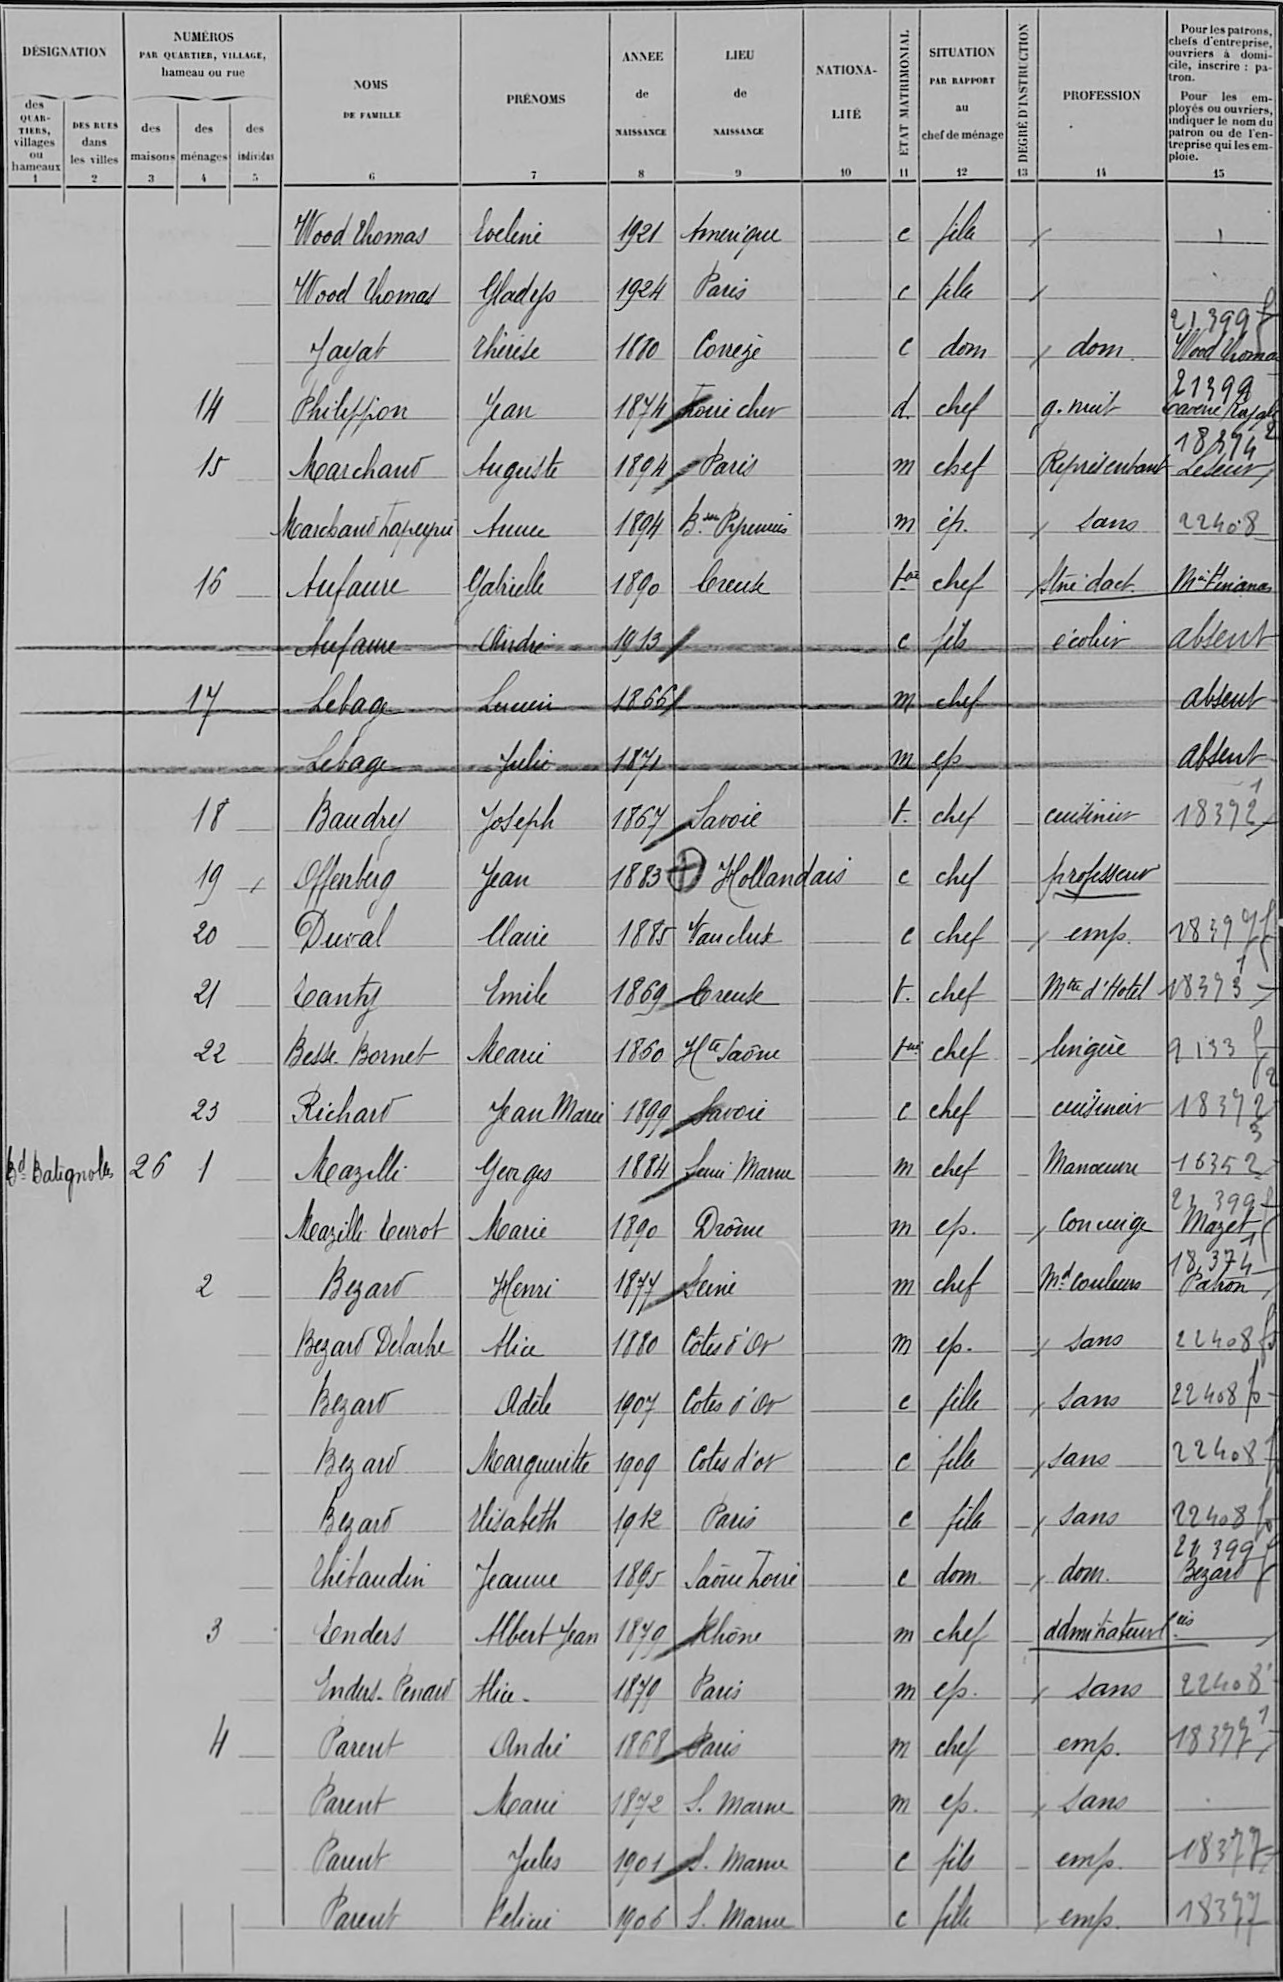Wood Thomas/Eveline/1921/Amerique/¤/C/fille/¤/¤/¤
Wood Thomas/Gladys/1924/Paris/¤/C/fille/¤/¤/¤
Jayat/Thérèse/1880/Corrèze/¤/C/dom/¤/dom/Wood Thomas 21399
Philippon/Jean/1874/Loire cher/¤/d/chef/¤/g nuit/Taverne Royale ?21399
Karchant/Auguste/1894/Paris/¤/m/chef/¤/représentant/Leseur ?18374
Karchant Lapeyre/Anne/1894/Bse Pyrennées/¤/m/ép /¤/sans/22408
Aufaure/Gabrielle/1890/Creuse/¤/Vve/chef/¤/Stén dact /Min Finances
Aufaure/André/1913/¤/¤/C/fils/¤/écolier/absent
Lebage/Lucien/1866/¤/¤/m/chef/¤/¤/absent
Lebage/Julie/1871/¤/¤/m/ep/¤/¤/absent
Baudry/Joseph/1867/Savoie/¤/V/chef/¤/cuisiner/18372
Offenberg/Jean/1883/Hollandais/¤/c/chef/¤/professeur/¤
Duval/Marie/1885/Vaucluse/¤/c/chef/¤/emp/183971
Canty/Emile/1869/Creuse/¤/V /chef/¤/Mtre d'hotel/18392
Besse Bornet/Marie/1860/Hte Saône/¤/Vve/chef/¤/lingère/9133
Richard/Jean Marie/1899/Savoie/¤/C/chef/¤/cuisinier/18372
keazelli/Georges/1884/Seine Marne/¤/m/chef/¤/manoeuvre/16352
keazelli Leurot/Marie/1890/Drôme/¤/m/ep /¤/concierge/Mazet ?23599
Bezard/Henri/1877/Seine/¤/m/chef/¤/md couleurs patron/?18374
Bezard Delaihe/Alice/1880/Cotes d'Or/¤/m/ep /¤/sans/22408
Bezard/Adèle/1907/Cotes d'Or/¤/c/fille/¤/sans/22408
Bezard/Marguerite/1909/Cotes d'Or/¤/c/fille/¤/sans/22408
Bezard/Elisabeth/1912/Paris/¤/c/fille/¤/sans/22408 22399
Chétaudin/Jeanne/1895/Saône Loire/¤/c/dom/¤/dom/Bezard ?21399
Lenders/Albert Jean/1879/Rhône/¤/m/chef/¤/administrateur/¤
Lenders Perraud/Alice/1879/Paris/¤/m/ep /¤/sans/22408
Parent/André/1868/Paris/¤/m/chef/¤/emp /18377
Parent/Marie/1872/S Maine/¤/m/ep /¤/sans/¤
Parent/Jules/1901/S Maine/¤/C/fils/¤/emp /18377
Parent/Felicie/1906/S Maine/¤/c/fille/¤/emp /18377
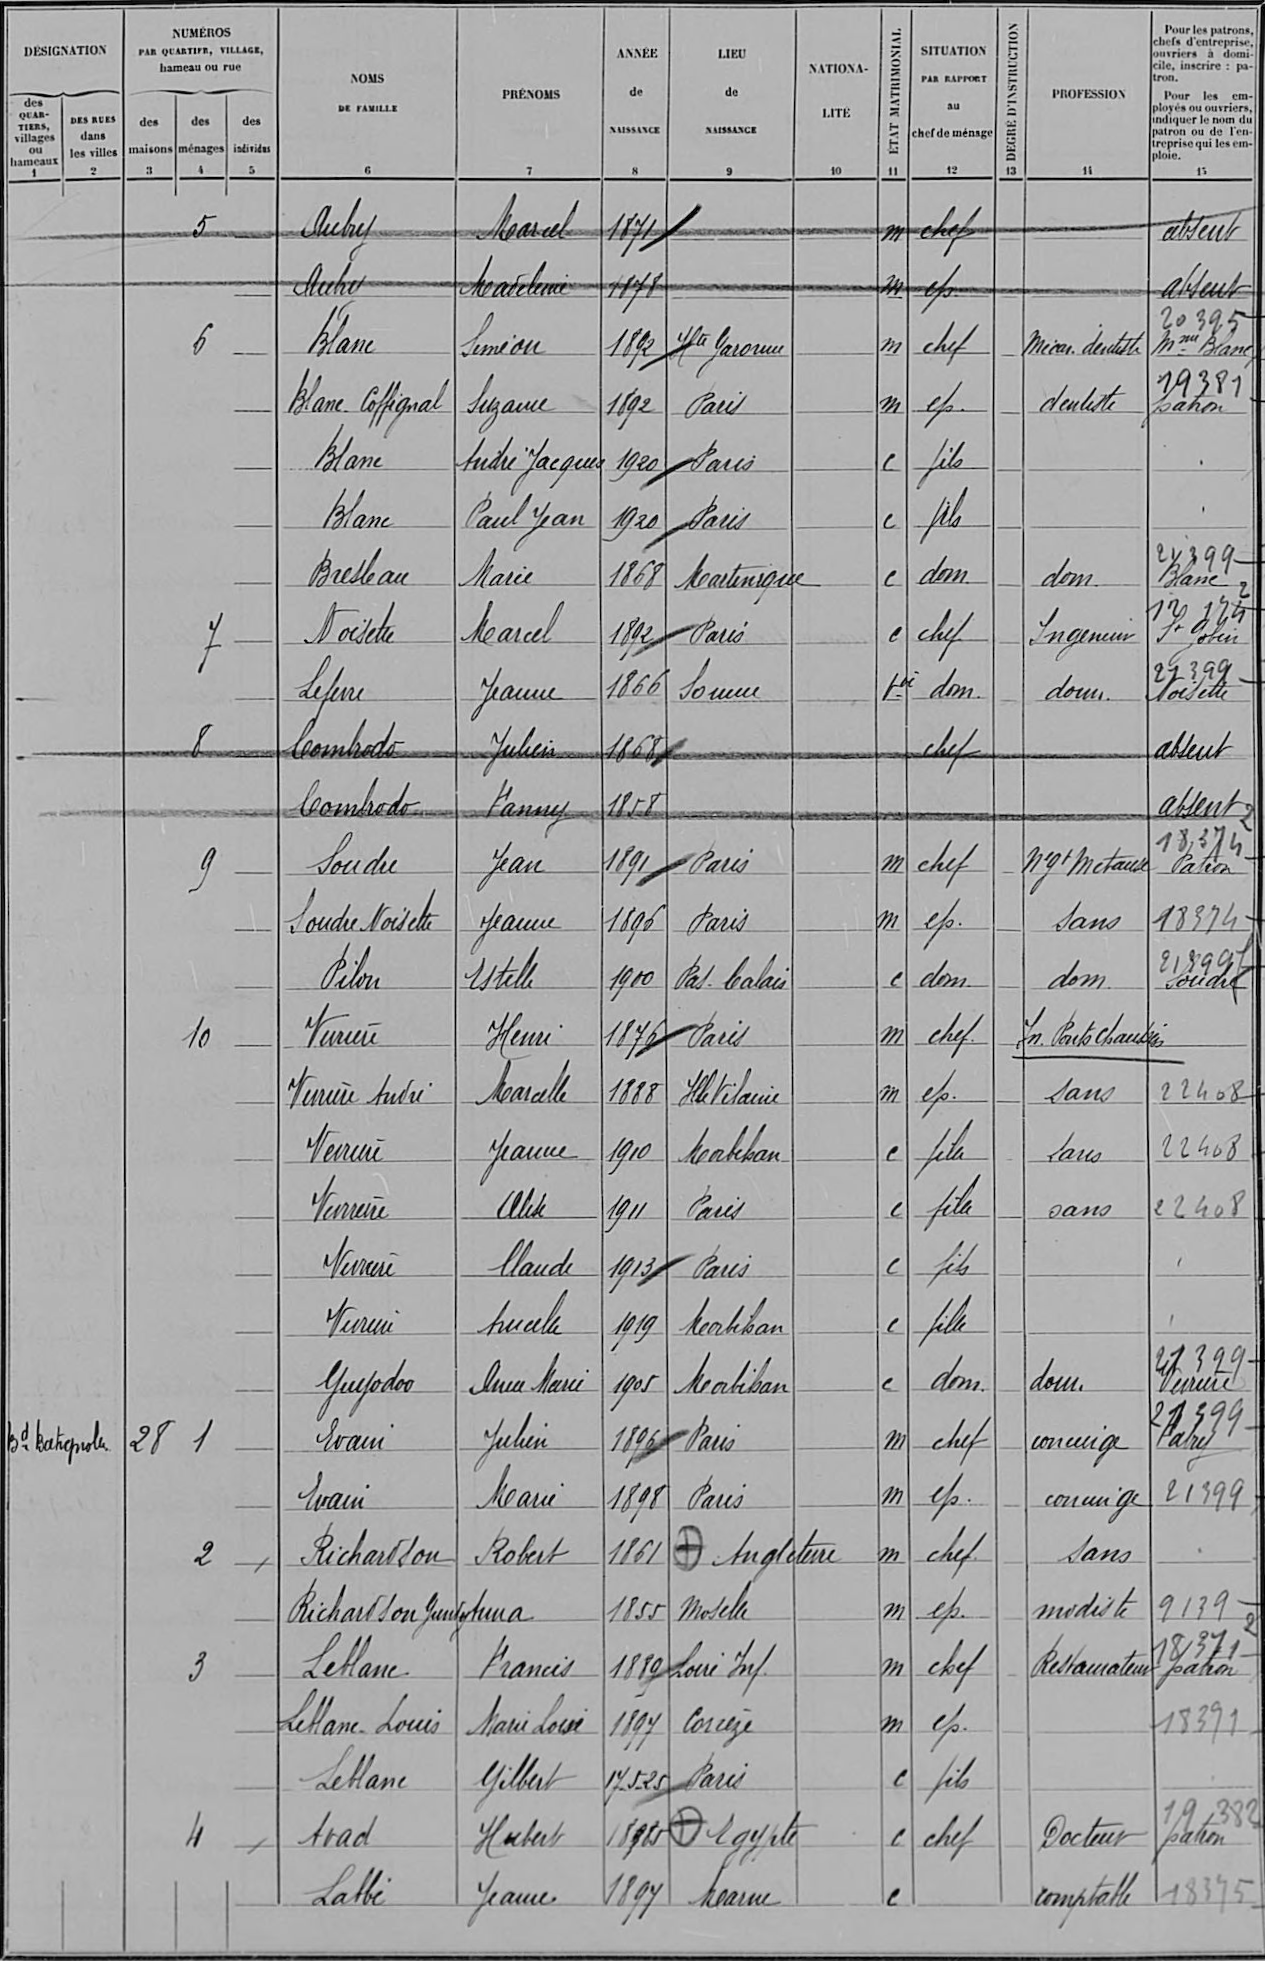Aubry/Marcel/1871/¤/¤/m/chef/¤/¤/absent
Aubry/Madeleine/1878/¤/¤/m/ep /¤/¤/absent
Blanc/Siméon/1892/Hte Garonne/¤/m/chef/¤/mécan dentiste/Mson Blanc ?20395
Blanc Coffignal/Suzanne/1892/Paris/¤/m/ep /¤/dentiste /patron ?19381
Blanc/André Jacques/1920/Paris/¤/C/fils/¤/¤/¤
Blanc/Paul Jean/1920/Paris/¤/C/fils/¤/¤/¤
Bresleau/Marie/1868/Martinique/¤/C/dom /¤/dom /Blanc ?24399
Noisette/Marcel/1892/Paris/¤/C/chef/¤/Ingénieur /St Gobin?121242
Lefevre/Jeanne/1866/Somme/¤/Vve/dom /¤/dom /Noisette ?21399
Combrodo/Julien/1868/¤/¤/¤/chef/¤/¤/absent
Combrodo/Fanny/1858/¤/¤/¤/¤/¤/¤/absent
Soudre/Jean/1891/paris/¤/m/chef/¤/Ngt metaux patron/?18374
Soudre Noisette/Jeanne/1896/Paris/¤/m/ep /¤/sans/18374
Pilon/Estelle/1900/Pas Calais/¤/c/dom /¤/dom /Soudre ?21399
Vuriere/Henri/1876/Paris/¤/m/chef/¤/In Ponts chaussées/¤
Vuriere André/Marcelle/1888/Ile et Vilaine/¤/m/ep /¤/sans/22408
Vuriere/Jeanne/1910/Morbihan/¤/c/fille/¤/sans/22408
Vuriere/Alise/1911/Paris/¤/c/fille/¤/sans/22408
Vuriere/Claude/1913/Paris/¤/c/fils/¤/¤/¤
Vuriere/Armelle/1919/Morbihan/¤/c/fille/¤/¤/¤
Guyodoo/Anne Marie/1905/Morbihan/¤/C/dom/¤/dom/Vurière ?21329
Evain/Julien/1896/Paris/¤/m/chef/¤/concierge/Patry ?21399
Evain/Marie/1898/Paris/¤/m/ep /¤/concierge/21399
Richardson/Robert/1861/Angleterre/¤/m/chef/¤/sans/¤
Richardson/Gunghuna/1855/Moselle/¤/m/ep /¤/modiste/9139
Leblanc/Francis/1889/Loire Inf/¤/m/chef/¤/Restaurateur/patron ?18371
Leblanc Louis/Marie Loise/1897/Corrèze/¤/m/ep /¤/¤/18391
Leblanc/Gilbert/19 5-25/Paris/¤/c/fils/¤/¤/¤
Trad/Hubert/1885/Egypte/¤/c/chef/¤/Docteur /patron ?19382
Labbé/Jeanne/1894/Marne/¤/c/¤/¤/comptable/18375